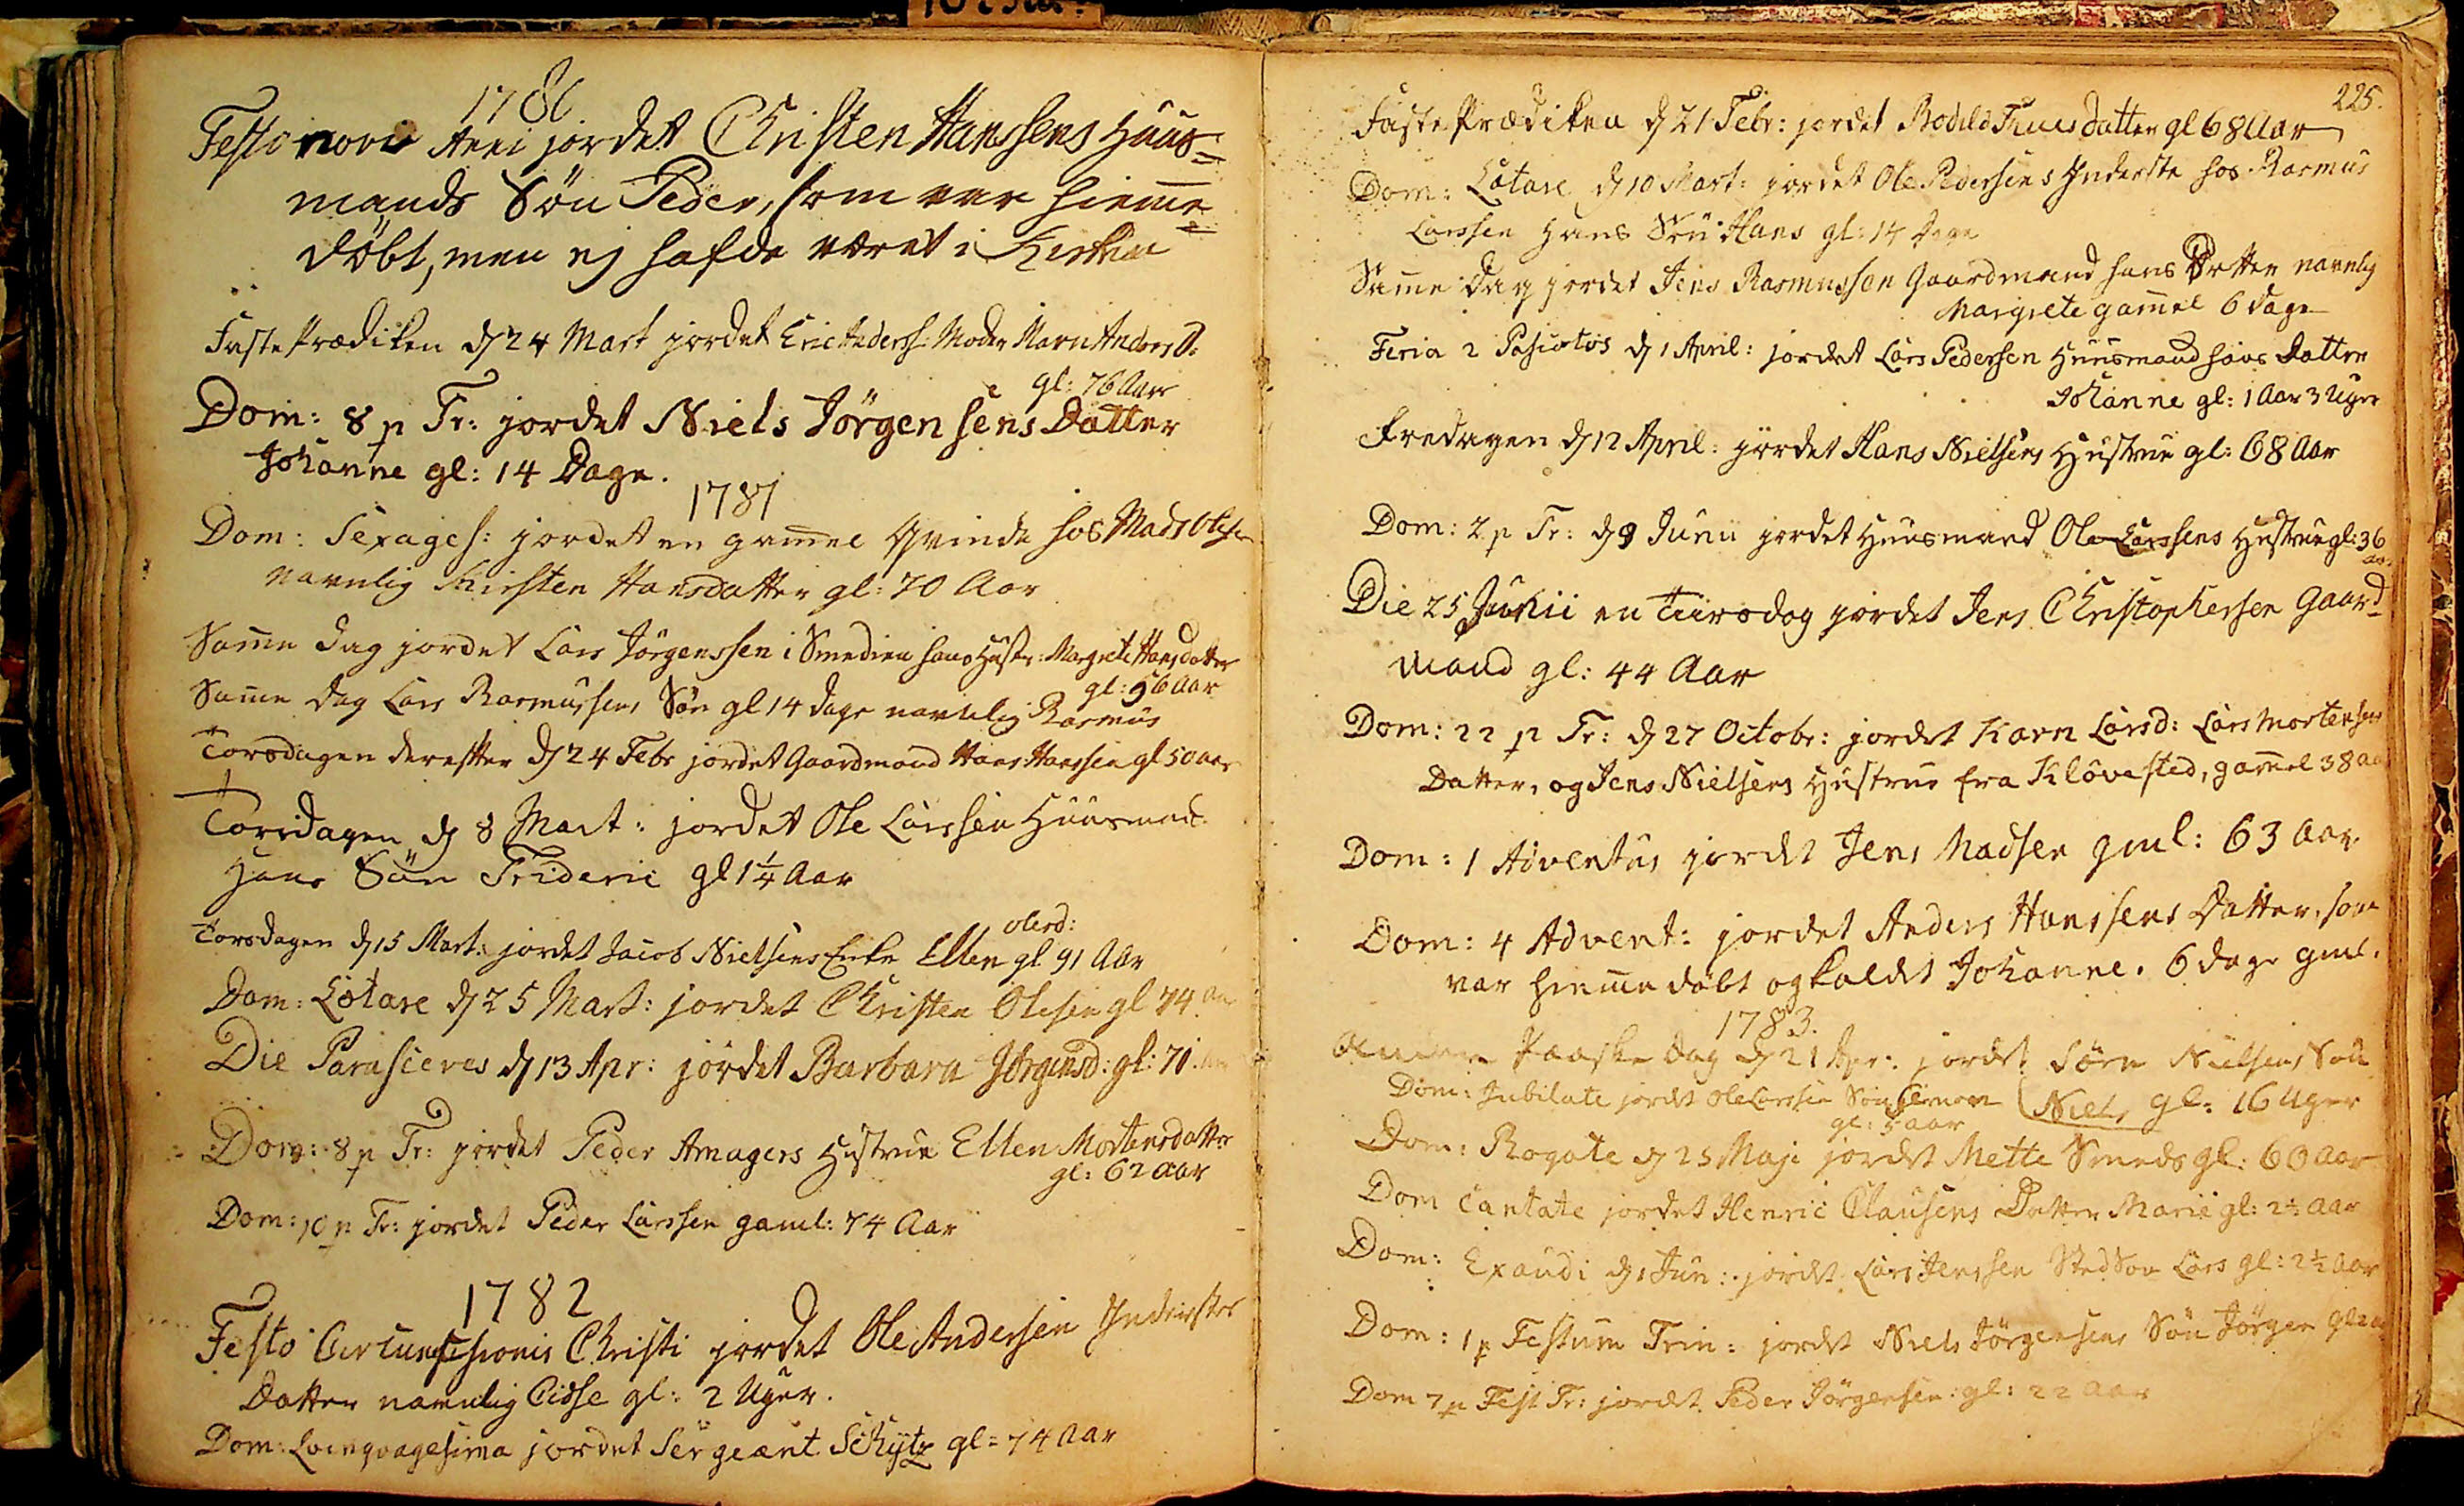1780.
Festo novi Anni jordet Christen Hanssens Huus¬
mands Søn Peder, som var hiemme¬
døbt, men ej hafde været i Kirken
Faste Prædiken d 24 Mart jordet Eric Anderss: Moder Marn Andersd: 
gl: 76 Aar
Dom: 8 p Tr: jordet Niels Jørgensens Datter 
Johanne gl: 14 Dage.
1781
Dom: Sexages: jordet en gammel Qvinde hos Mads Olesen
navnlig Kirsten Hansdatter gl: 70 Aar
Samme Dag jordet Lars Jørgenssen i Smedien hans Hustr: Margrete Hansdatter
gl: 56 Aar
Samme Dag Lars Rasmussens Søn gl 14 dage navnlig Rasmus
Torsdagen derefter d 24 Febr jordet Gaardmand Hans Hanssen gl 50 Aar
Torsdagen d 8 Mart: jordet Ole Larssen Huusmand
Hans Søn Frideric gl 1¼ Aar
Olesd:
Torsdagen d 15 Mart: jordet Jacob Nielsens Enke Ellen gl 91 Aar
Dom: Lætare d 25 Mart: jordet Christen Olesen gl 74 Aar
Die Parasceves d 13 Apr: jordet Barbara Jørgensd: gl: 71 Aar
Dom: 8 p Tr: jordet Peder Amagers Hustrue Ellen Mortensdatter   
gl: 62 Aar
Dom: 10 p Tr: jordet Peder Larssen gaml: 74 Aar
1782
Festo Circumschionis Christi jordet Ole Andersen Inderstes
Datter navnlig Cidse gl: 2 Uger
Dom: Qvinqvagesima jordet Sergeant Schytz gl: 74 Aar
225.
Faste Prædiken d 21 Febr: jordet Bodild Thuesdatter gl 68 Aar
Dom: Lætare d 10 Mart: jordet Ole Pedersens Inderste hos Rasmus
Larssen Hans Søn Hans gl: 14 Dage
Samme Dag jordet Jens Rasmussen Gaardmand hans Datter navnlig
Margrete gammel 6 dage
Feria 2 Pascatos d 1 April: jordet Lars Pedersen Huusmand hans Datter
Johanne gl: 1 Aar 3 Uger
Fredagen d 12 April: jordet Hans Nielsens Hustrue gl: 68 Aar
Dom: 2. p Tr: d 9 Junii jordet Huusmand Ole Larssens Hustrue 36
Aar
Die 25 Junii en Tiirsdag jordet Jens Christophersen Gaard¬
mand gl: 44 Aar
Dom: 22 p Tr: d 27 Octobr: jordet Karn Larsd: Lars Mortensens
Datter, og Jens Nielsens Hustrue fra Kløvested, gammel 38 Aar
Dom: 1 Adventus jordt Jens Madsen gml: 63 Aar
Dom: 4 Advent: jordet Anders Hanssens Datter, som
var hiemmedøbt og kaldet Johanne. 6 dage gml.
1783.
Anden Paaske Dag d 21 Apr: jordt Sørn Nielsens Søn
Niels gl: 16 Uger
Dom: Jubilate jordet Ole Larssen Søn Simon             
gl: 5 Aar
Dom: Rogate d 25 Maji jordt Mette Smeds gl: 60 Aar
Dom: Cantate jordet Henric Clausens Datter Marie gl: 2½ Aar
Dom: Exaudi d 1 Jun: jordt Lars Jenssen StedSøn Lars gl: 2½ Aar
Dom: 1 p Festum Trin: jordt Niels Jørgensens Søn Jørgen gl 2 Aar
Dom 7 p Fest Tr: jordt Peder Jørgensen. gl: 22 Aar